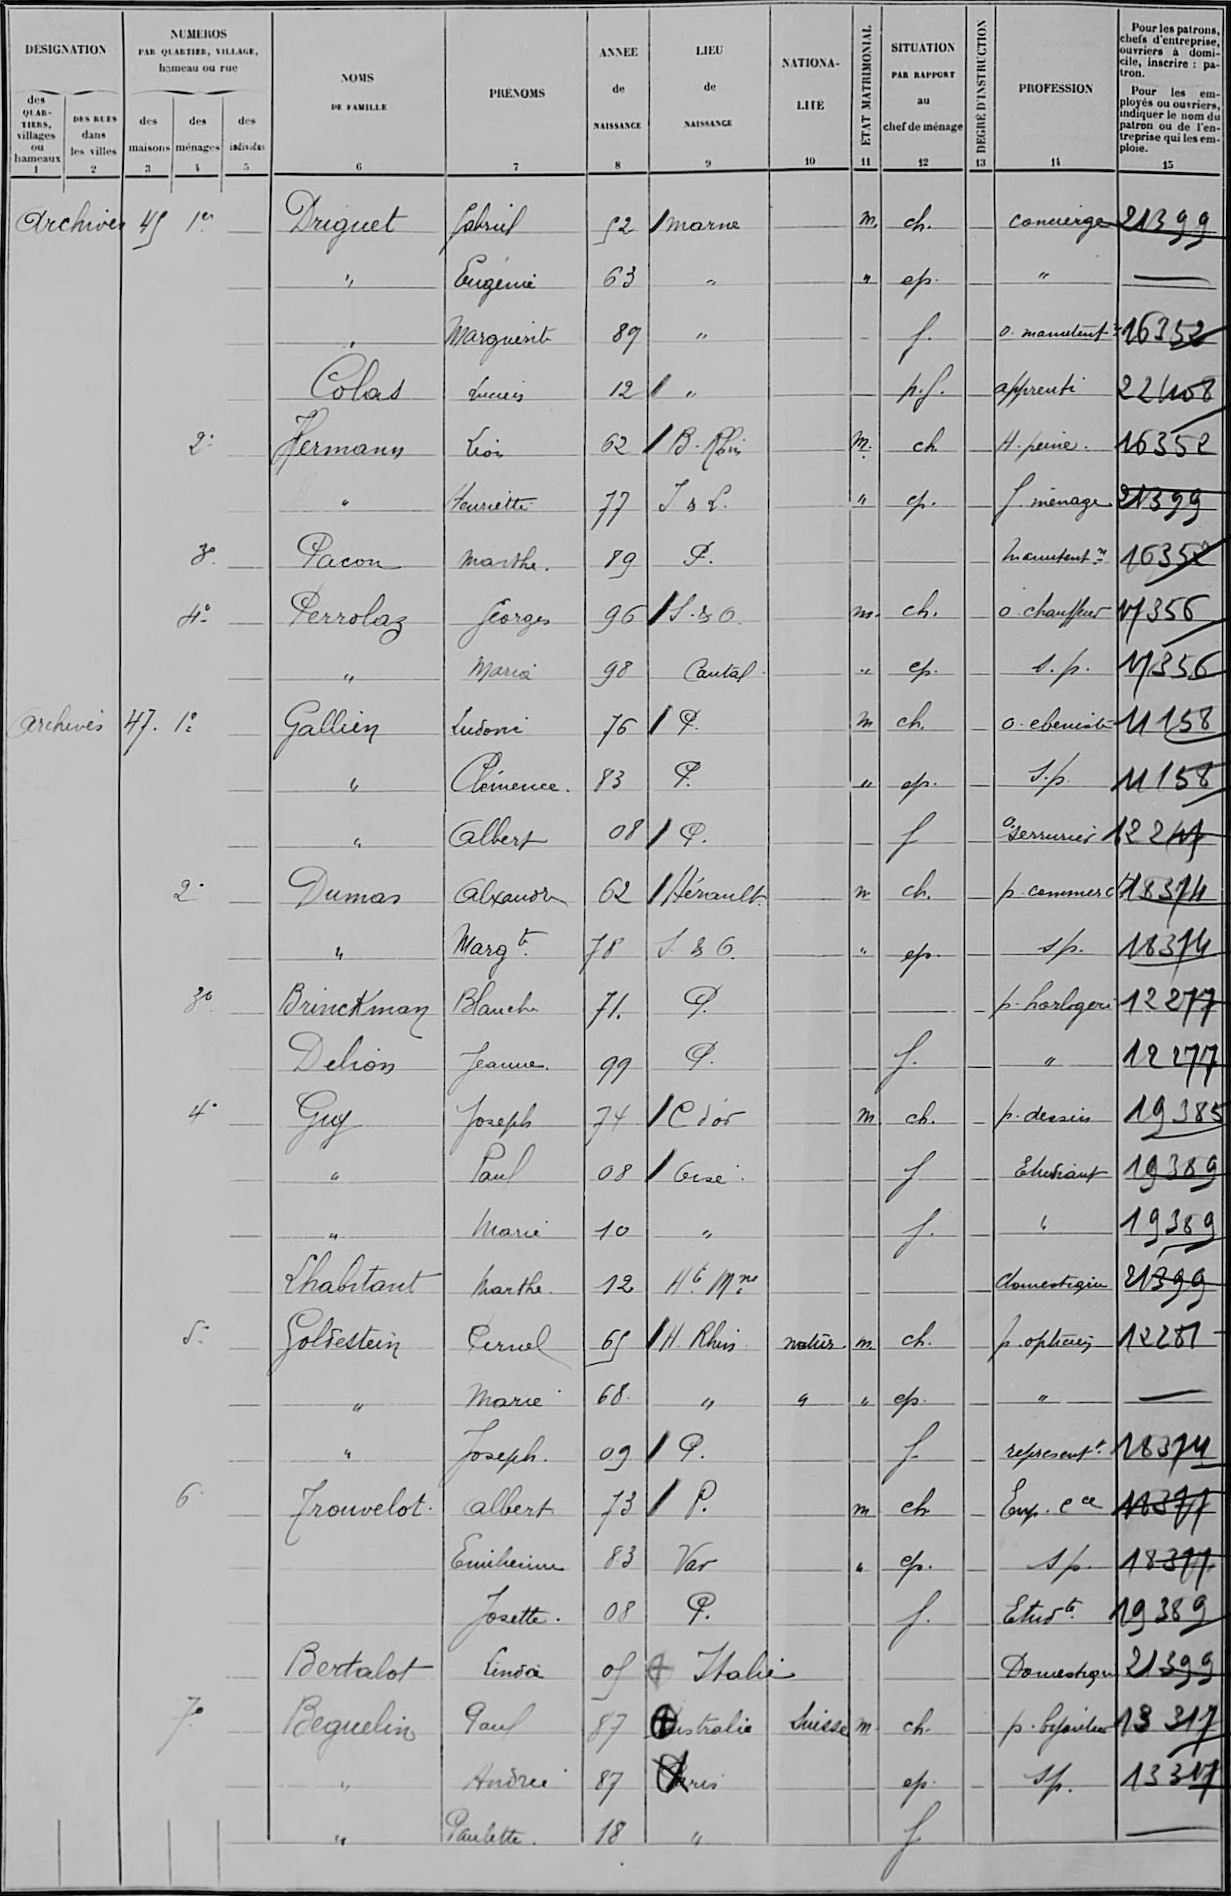Driguet/Gabriel/52/Marne/¤/M /ch /¤/concierge/21399
"/Eugénie/63/"/¤/"/ep /¤/"/¤
"/Marguerite/89/"/¤/¤/f /¤/o manutent /16352
Colas/Lucien/12/"/¤/¤/p f /¤/apprenti/22408
Hermann/L2on/62/B Rhin/¤/M /ch/¤/H peine/16352
"/Henriette/77/I & L /¤/"/ep /¤/f ménage/21399
Pacon/Marthe/89/P /¤/¤/¤/¤/Manutentre/16352
Perrolaz/Georges/96/S & O /¤/M /ch /¤/o chauffeur/17356
"/Maria/98/Cantal/¤/"/ep /¤/s p /17356
Gallien/Ludovic/76/P /¤/M/ch /¤/o ebeniste/11158
"/Clémence/83/P /¤/"/ep /¤/S p/11158
"/Albert/08/P /¤/¤/f/¤/o serrurier/12247
Dumas/Alexandre/62/Hérault/¤/m/ch /¤/p commerce/18374
¤/Margte/78/S & O /¤/"/ep /¤/Sp /18374
Brinckman/Blanche/71/P /¤/¤/¤/¤/p horloger/12277
Delion/Jeanne/99/P /¤/¤/f /¤/"/12277
Guy/Joseph/74/C d'or/¤/M/ch /¤/p dessin/19385
"/Paul/08/Oise/¤/¤/f/¤/Etudiant/19389
"/Marie/10/"/¤/¤/f /¤/"/19389
Lhabitant/Marthe/12/Hte Mne/¤/¤/¤/¤/domestique/21399
Goldestein/Cernel/65/H Rhin/natur/m/ch /¤/p opticien/12287
"/Marie/68/"/"/"/ep /¤/"/¤
"/Joseph/09/P /¤/¤/f /¤/représentt/18374
Trouvelot/albert/73/P /¤/m/ch /¤/Emp Cce/18377
¤/Emilienne/83/Var/¤/"/ep /¤/S p /18377
¤/Josette/08/P /¤/¤/f /¤/Etudte/19389
Bertalot/Linda/05/Italie/¤/¤/¤/¤/Domestique/21399
Beguelin/Paul/87/Australie/SUisse/m /ch /¤/p bijoutier/13317
"/Andrée/87/Paris/¤/¤/ep /¤/Sp /13317
"/Paulette/18/"/¤/¤/f /¤/¤/¤
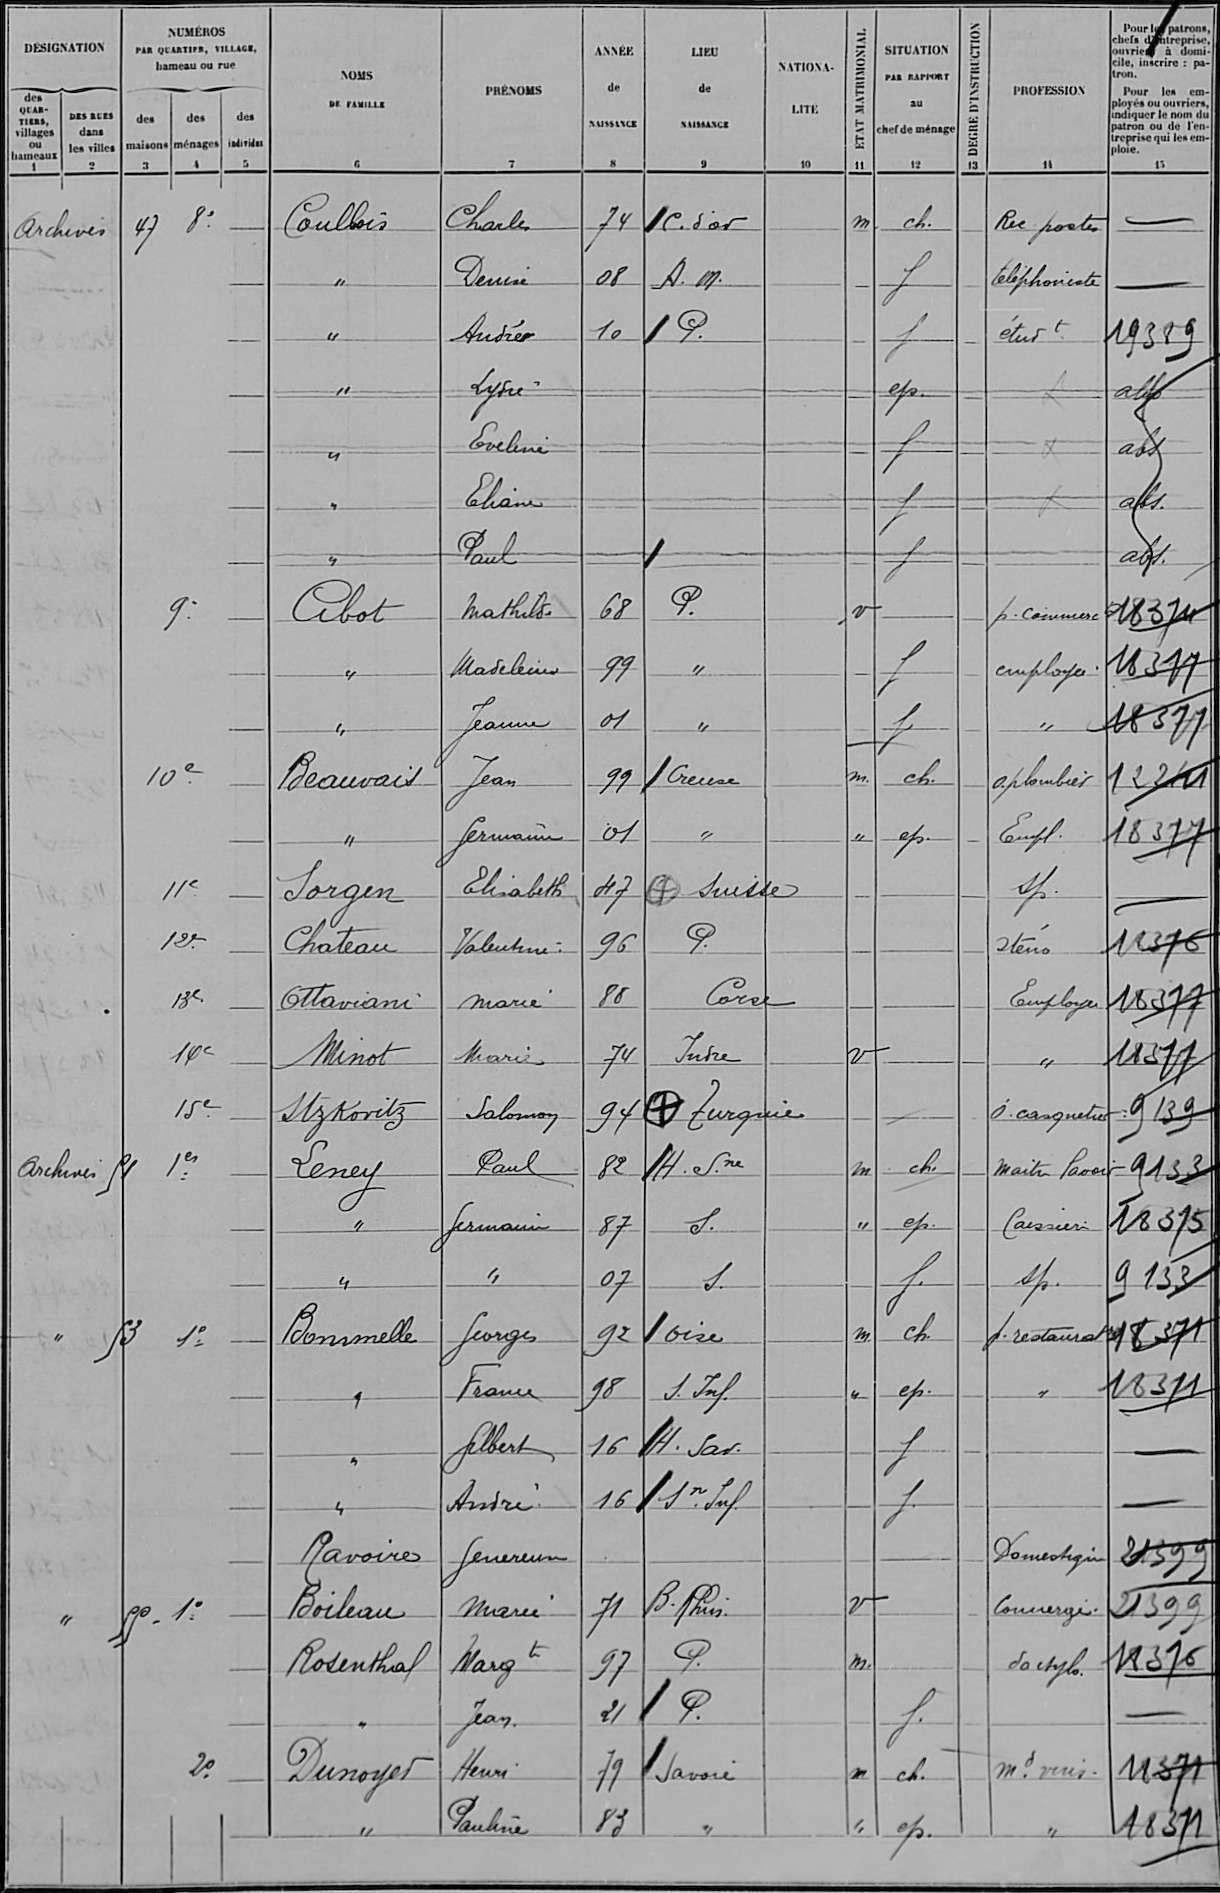Coulbois/Charles/74/C d'or/¤/m/ch /¤/Rec Postes/¤
"/Denise/08/A M /¤/¤/f/¤/telephoniste/¤
"/André/10/P /¤/¤/f/¤/étudt/19389
"/Lydie/¤/¤/¤/¤/ep /¤/¤/abs
"/Eveline/¤/¤/¤/¤/f/¤/¤/abs
"/Eliane/¤/¤/¤/¤/f/¤/¤/abs
"/Paul/¤/¤/¤/¤/f/¤/¤/abs
Cibot/Mathilde/68/P /¤/v/¤/¤/p commerce/18374
"/Madeleine/99/"/¤/¤/f/¤/employée/18377
"/Jeanne/01/"/¤/¤/f /¤/"/18377
Beauvais/Jean/99/Creuse/¤/m/ch /¤/o plombier/12241
¤/Germaine/01/"/¤/"/ep /¤/Empl /18377
Sorgen/Elisabeth/47/Suisse/¤/¤/¤/¤/Sp /¤
Chateau/Valentine/96/P /¤/¤/¤/¤/Sténo/18376
Ottaviani/Marie/88/Corse/¤/¤/¤/¤/Employée/18377
Minot/Marie/74/Indre/¤/V/¤/¤/"/18377
Stzkovitz/Salomon/9/Turquie/¤/¤/¤/¤/o casquetier/9139
Leney/Paul/82/H Sne/¤/M/ch /¤/Maître lavoir/9133
"/Germaine/87/S /¤/"/ep /¤/Caissier/18375
"/"/07/S /¤/¤/f /¤/Sp /9133
Bonimelle/Georges/92/Oise/¤/m/ch /¤/p restauratr/18371
"/France/98/S Inf /¤/"/ep /¤/"/18371
"/Gilbert/16/H Sav /¤/¤/f/¤/¤/¤
"/André/16/Sn Inf /¤/¤/f /¤/¤/¤
Ravoire/Genereuse/¤/¤/¤/¤/¤/¤/Domestique/21399
Boileau/Marie/71/B Rhin/¤/v/¤/¤/Concierge/21399
Rosenthal/Margte/97/P /¤/m /¤/¤/dactylo/18376
"/Jean/21/P /¤/¤/f /¤/¤/¤
Duniyer/Henri/79/Savoie/¤/m/ch /¤/md vins/18371
"/Pauline/83/"/¤/"/ep /¤/"/18371